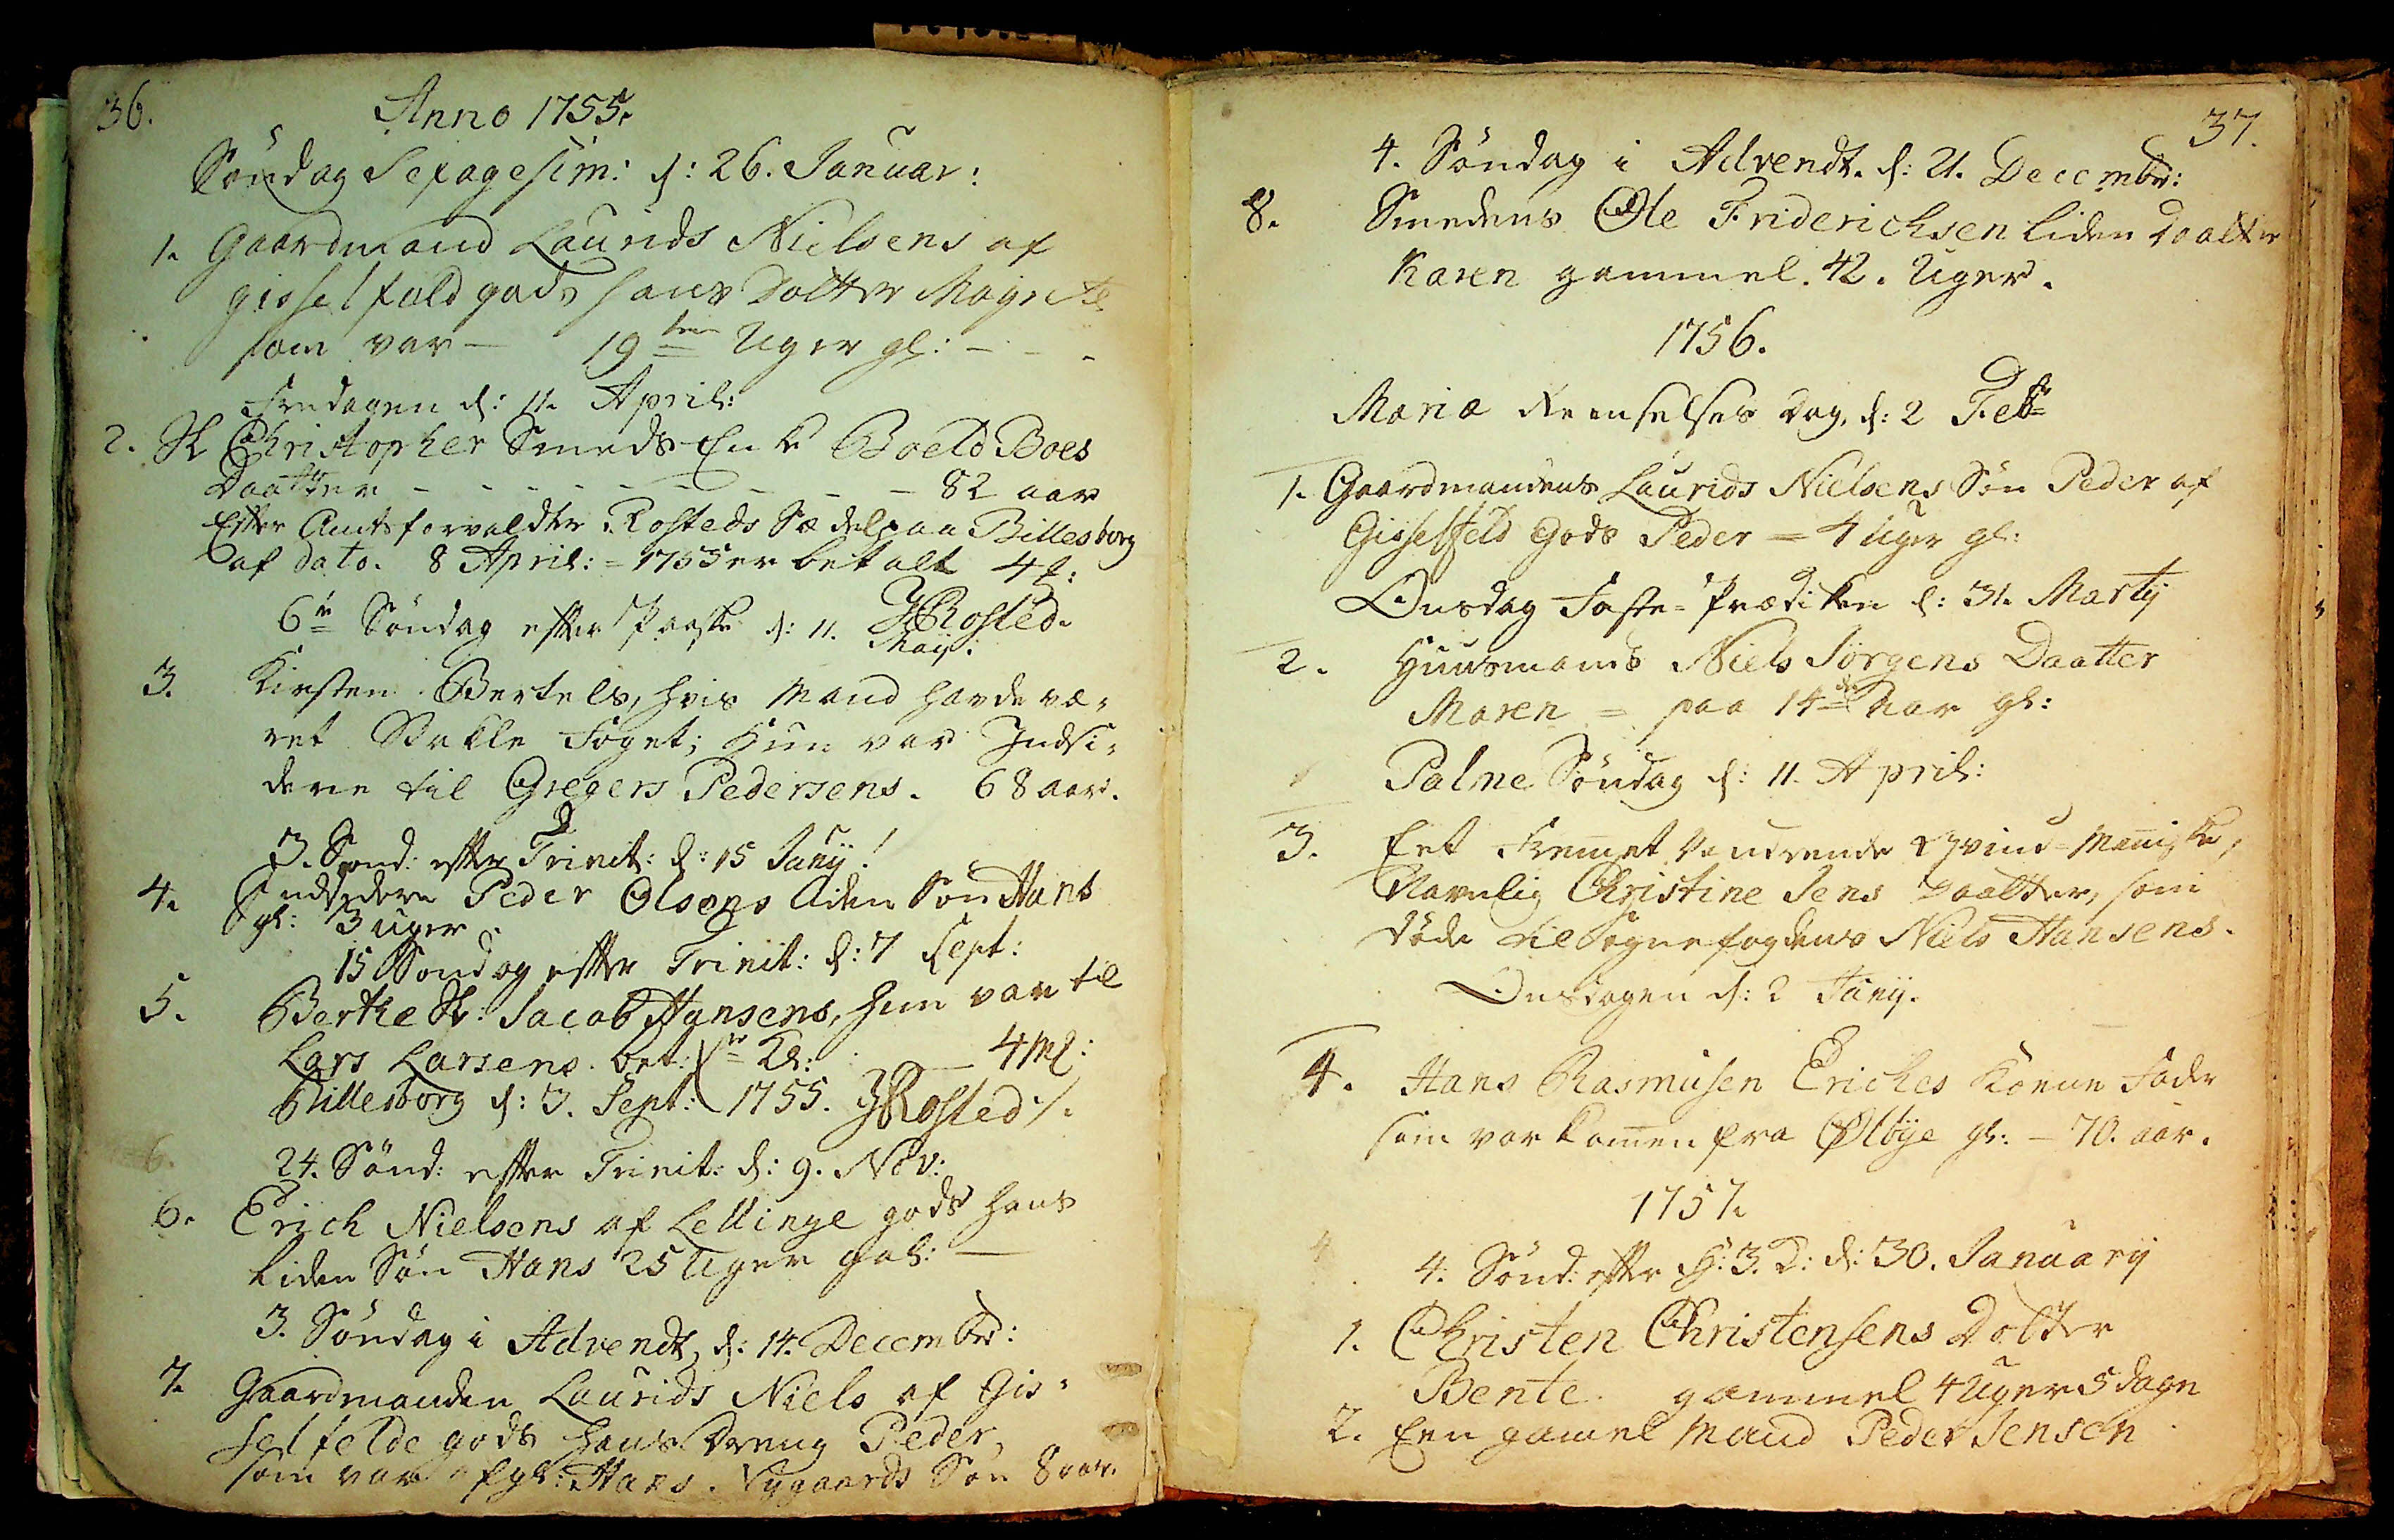36.
Anno 1755.
Søndag Sexagesim: d: 26 Januar:
1. Gaardmand Laurids Nielsens af
Gisselfeld Gods hans Datter Magrete
som var 
19ten Uger gl:
Fredagen d: 11. April:
2. Sl Christopher Smeds Enke Boeld Boes
Daatter 
82 Aar
Efter Amtsforvaldter Rosteds Sædel paa Billesborg
af dato. 8 April: =1755 er betalt 4 M:
JRosted.
6te Søndag efter Paaske d: 11. Maij.
3. Kirsten Bertels, hvis Mand havde væ¬
ret Skakle## Foget; Hun var Indsi¬
dere til Gregers Pedersens. 68 Aar.
3. Sønd: efter Trinit: d: 15 Junij !
4. Indsiddere Peder Olsens liden Søn Hans
gl: 3 Uger.
15 Søndag efter Trinit: d: 7 Sept:
5. Berthe Sl: Jacob Hansens, hun var til
Lars Larsens. bet: Xv## Kl:
4 Mk:
Billesborg d: 3. Sept: 1755. JRosted ./.
6. 24. Sønd: efter Trinit: d: 9. Nov:
6. Erich Nielsens af Lellinge Gods hans
liden Søn Hans 25 Uger gal: -
3. Søndag i Advendt, d: 14. Decembr:
7. Gaardmanden Laurids Niels af Gis¬
felde Gods hans Dreng Peder
som var afgl: Hans Nygaards Søn 8 aar.
37.
4. Søndag i Advendt. d: 21. Decembr:
8. Smedens Ole Friderichsen liden Daatter
Karen gammel. 42. Uger.
1756.
Mariæ Reenselses Dag. d: 2 Febr
1. Gaardmandens Laurids Nielsens Søn Peder af
Gisselfeld Gods Peder = 4 Uger gl:
Onsdag Faste=Prædiken d: 31. Martij
2. Huusmand Niels Jørgens Daatter
Maren = paa 14de Aar gl:
Palme Søndag d: 11. April:
3. Eet Fremmet vandrende Qvind=Menniske,
Navnlig Christine Jens Daatter, som
døde til Sognefogdens Niels Hansens.
Onsdagen d: 2 Junij.
4. Hans Rasmusen Eriches Koene Fadr
som var kommen fra Ølbye gl: - 70. Aar.
1757.
4. Sønd: efter H: 3. K: d: 30. Januarij
1. Christen Christensens Dotter
Bente. gammel 4 Uger 5 dage
2. Een gamel Mand Peder Jensen
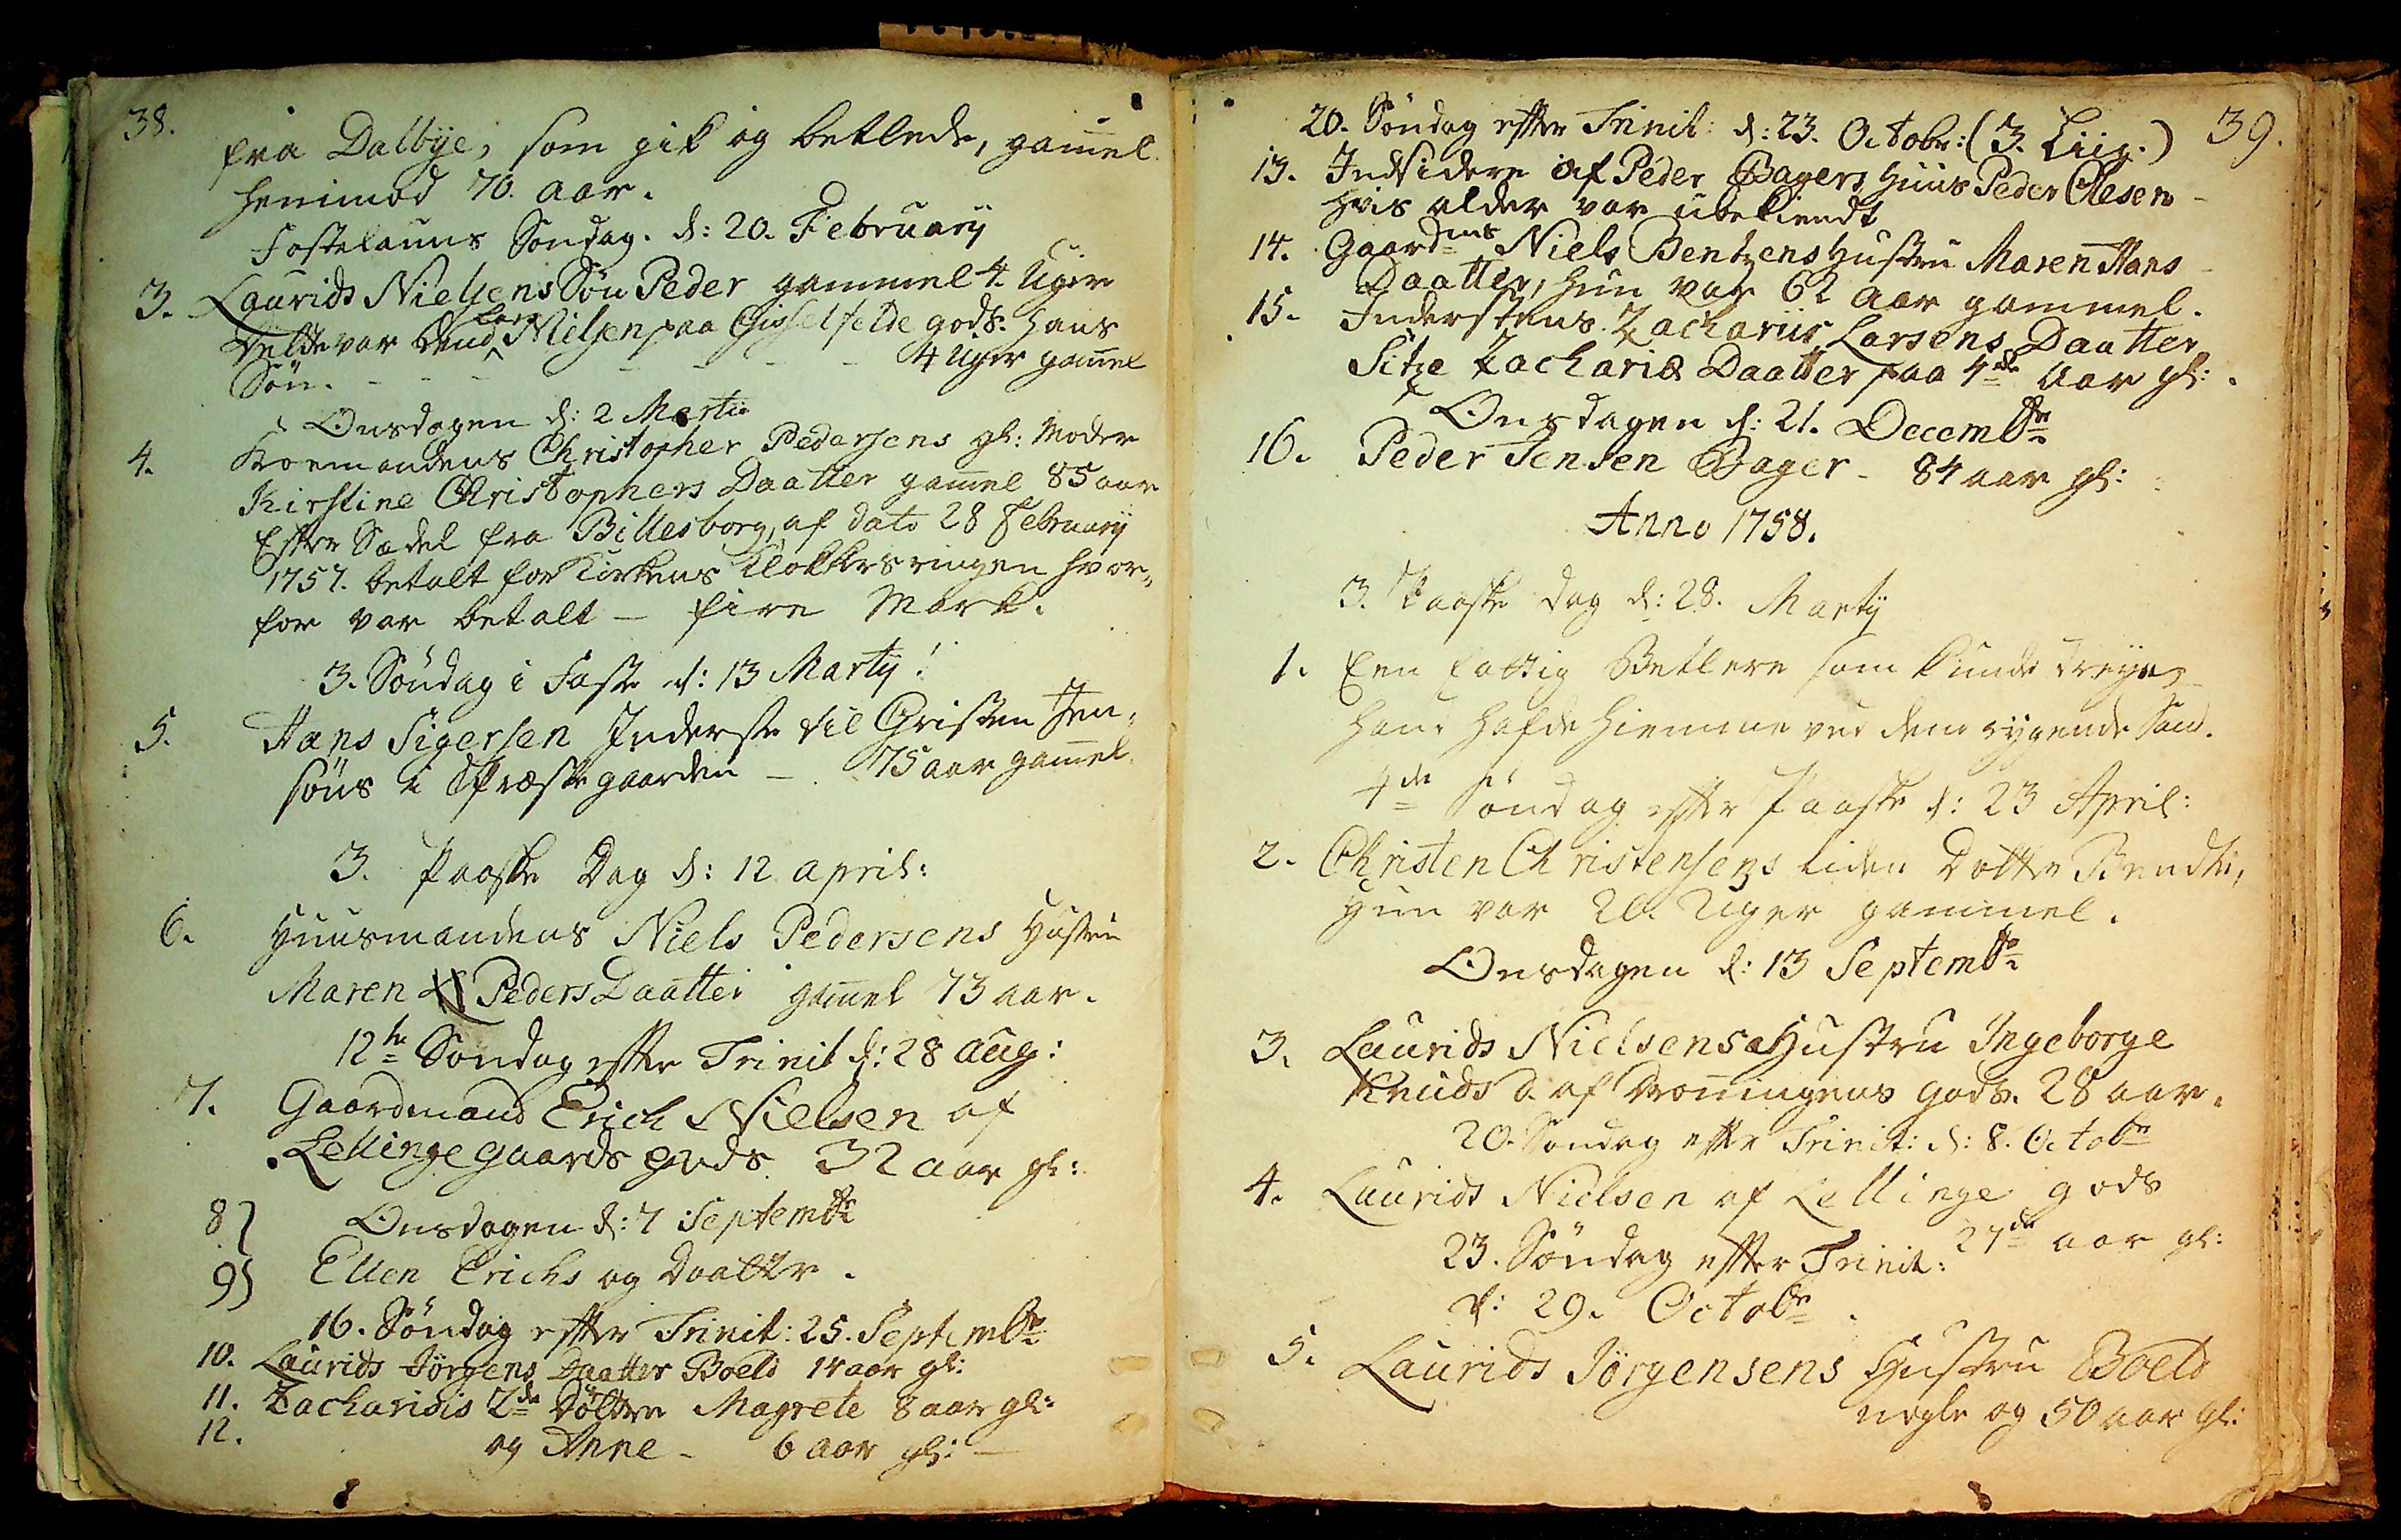38.
fra Dalbye, som gik og betlede, gammel
henimod 70. Aar.
Fastelauns Søndag. d: 20. Februarij
3. Laurids Nielsens Søn Peder gammel 4. Uger
Lars
Dette var dend Nielsen paa Gisselfelde Gods. hans
Søn.
4 Uger gammel
Onsdagen d: 2 Martii
4. Kroemandens Christopher Pedersens gl: Moder
Kirstine Christophers Daatter gammel 85 Aar
Efter Sædel fra Billesborg, af dato 28 Februarij
1757. betalt fra Kirkens Klokkes ringen hvor¬
for var betalt - fire Mark.
3. Søndag i Faste d: 13 Martij !
5. Hans Sigersen Inderste til Christen Jen¬
søns i Præstegaarden -75 Aar gammel
3. Paaske Dag d: 12 April:
6. Huusmandens Niels Pedersens Hustru
Maren L Peders Daatter gammel 73 Aar.
12te Søndag efter Trinit d: 28 Aug:
7. Gaardmand Erich Nielsen af
Lellingegaards Gods. 32 Aar gl:
8
9
Onsdagen d: 7 Septembr
Ellen Erichs og Daatter.
16. Søndag efter Trinit: 25. Septembr
10. Laurids Jørgens daatter Boeld 14 aar gl:
11. Zacharisis 2de Døttre Magrete 8 aar gl:
12. 
og Anne -6 aar gl:
39.
20. Søndag efter Trinit: d: 23. Octobr: (3. Liig.)
13. Indsidere af Peder Bagers Huus Peder Olesen
hvis Alder var ubekiendt
14. Gaardms Niels Bentzens Hustru Maren Hans
Daatter, hun var 62 Aar gammel.
15. Inderstens Zachariis Larsens Daatter
Sitze Zachariæ Daatter paa 4de Aar gl:
Onsdagen d: 21. Decembr
16. Peder Jensen Bager - 84 Aar gl:
Anno 1758.
3. Paaske Dag d: 28. Martij
1. Een fattig Betlere som kunde dreye,
hand havde hiemme ved den rygende## Søn##.
4de Søndag efter Paaske d: 23 April:
2. Christen Christensens liden Datter Bendte,
Hun var 20. Uger gammel.
Onsdagen d: 13 Septembr
3. Laurids Nielsens Hustru Ingeborge
Knudsd: af Dronningens Gods. 28 Aar.
20. Søndag efter Trinit: d: 8. Octobr
4. Laurids Nielsen af Lellinge Gods
27 Aar gl:
23. Søndag efter Trinit:
d. 29. Octobr.
5. Laurids Jørgensens Hustru Boeld
nogle og 50 Aar gl: